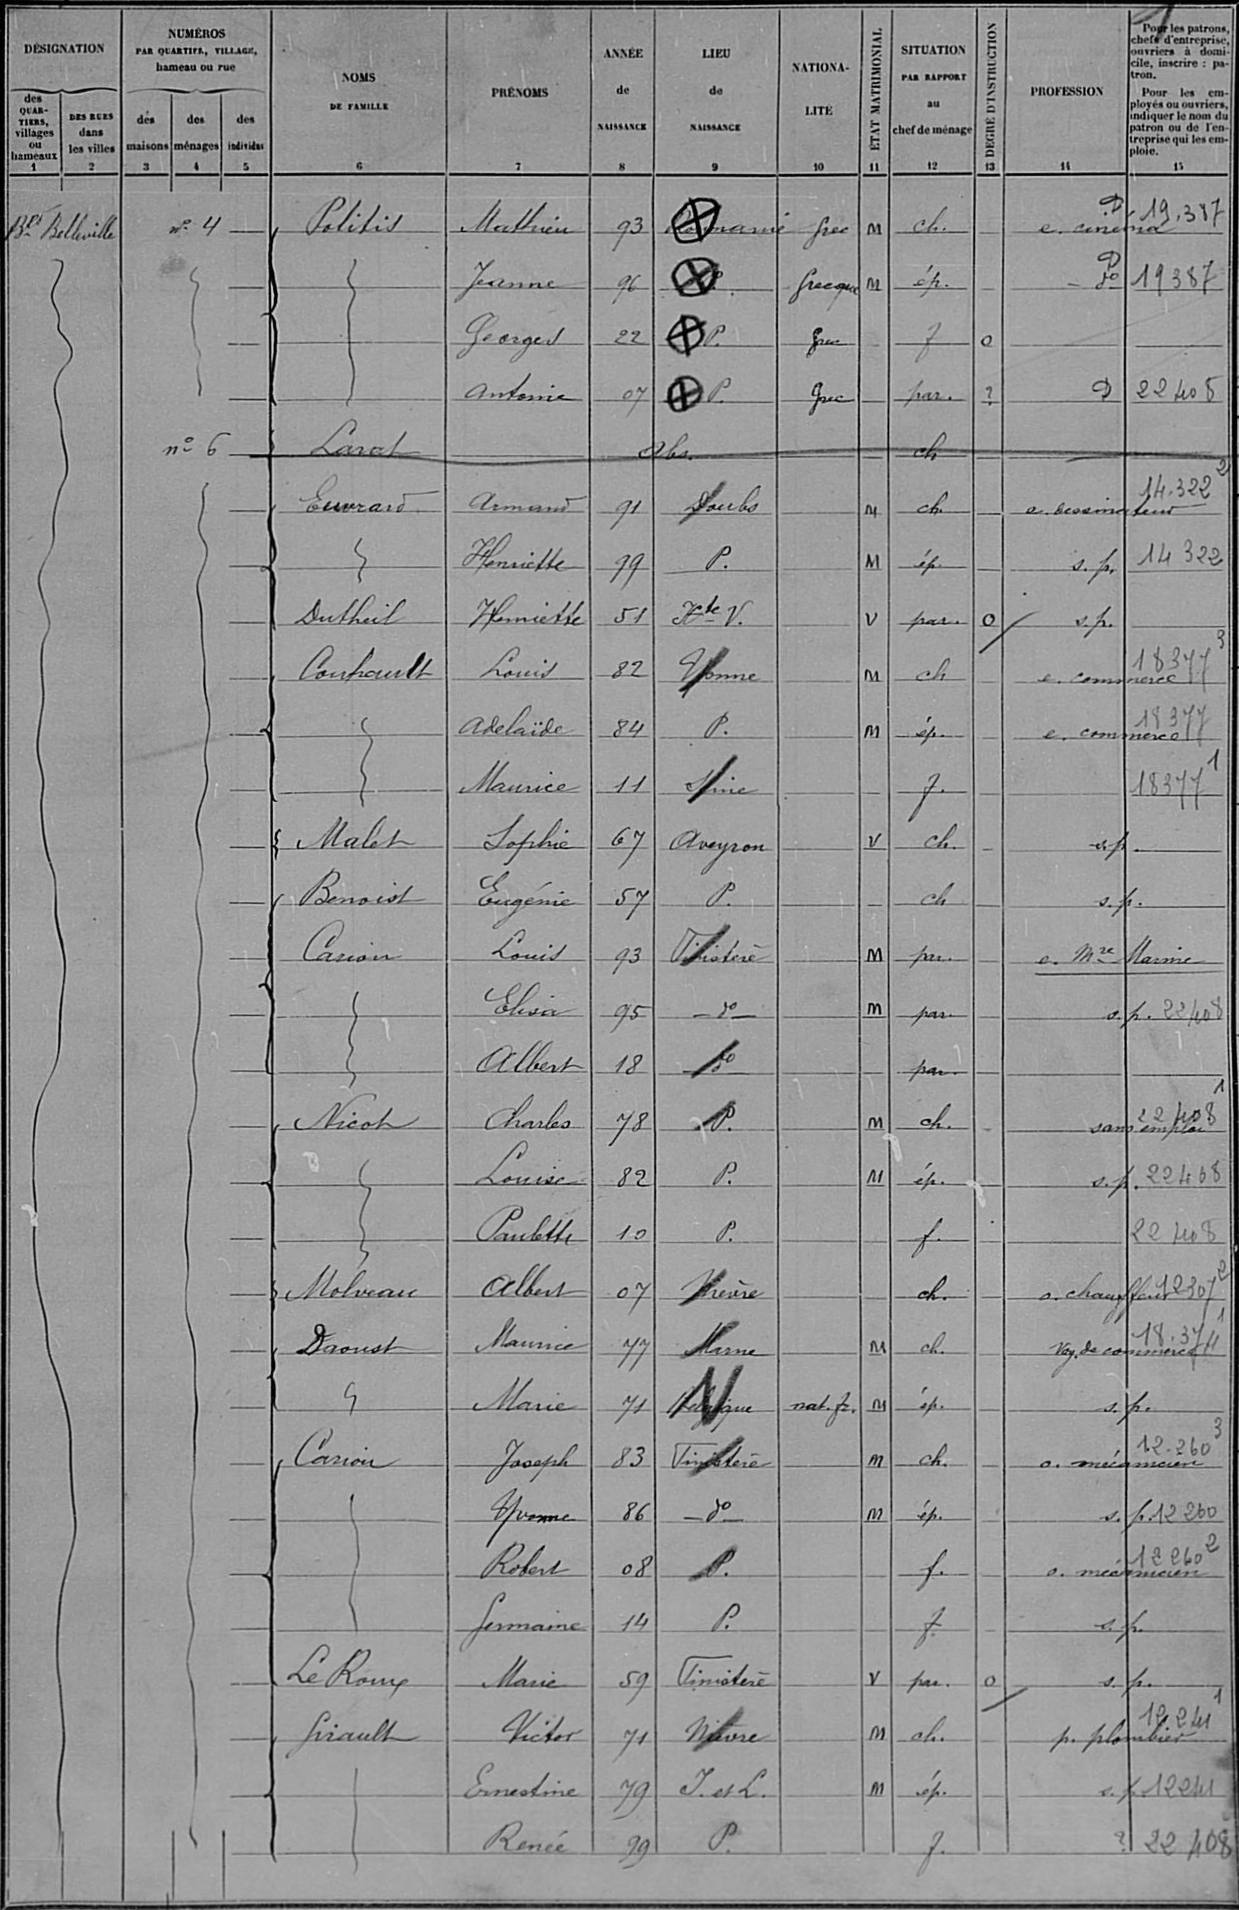Politis/Mathieu/93/Roumanie/grec/M/ch /¤/e cinéma/¤
¤/Jeanne/96/P /grecque/M/ép /¤/d°/19387
¤/Georges/22/P /grec/¤/f/o/¤/¤
¤/Antoine/07/P /grec/¤/par /¤/D/22408
Larat/¤/abs /¤/¤/¤/ch/¤/¤/¤
Euvrard/Armand/91/Doubs/¤/M/ch/¤/a dessinateur/¤
¤/Henriette/99/P /¤/M/ép /¤/s p/14322
Dutheil/Henriette/51/Hte V /¤/V/par /O/s p /¤
Couhault/Louis/82/Yonne/¤/M/ch/¤/e commerce/¤
¤/Adelaïde/84/P /¤/M/ép /¤/e commerce/¤
¤/Maurice/11/Seine/¤/¤/f /¤/¤/18377
Malet/Sophie/67/Aveyron/¤/V/ch/¤/s p /¤
Benoist/Eugénie/57/P /¤/¤/ch/¤/s p /¤
Carion/Louis/93/Finistère/¤/M/par /¤/e Mre Marine/¤
¤/Elisa/95/d°/¤/M/par /¤/s p /22408
¤/Albert/18/d°/¤/¤/par /¤/¤/¤
Nicoh/Charles/78/P /¤/M/ch /¤/sans emploi/¤
¤/Louise/82/P /¤/M/ép /¤/s p /22408
¤/Paulette/10/P /¤/¤/f /¤/¤/22408
Molvearc/Albert/07/Nièvre/¤/¤/ch /¤/o chauffeur/12307
Daoust/Maurice/77/Marne/¤/M/ch /¤/voy de commerce/¤
¤/Marie/71/Belgique/nat fr /M/ép/¤/s p /¤
Cariou/Joseph/83/Finistère/¤/M/ch /¤/o mécanicien/¤
¤/Yvonne/86/d°/¤/m/ép /¤/s p /12260
¤/Robert/08/P /¤/¤/f /¤/o mécanicien/¤
¤/Germaine/14/P /¤/¤/f/¤/s p /¤
Le Roux/Marie/59/Finistère/¤/V/par /o/s p /¤
Girault/Victor/71/Nièvre/¤/M/ch /¤/p plombier/¤
¤/Ernestine/79/I et L /¤/M/ép /¤/s p /12241
¤/Renée/99/P /¤/¤/f /¤/¤/22408
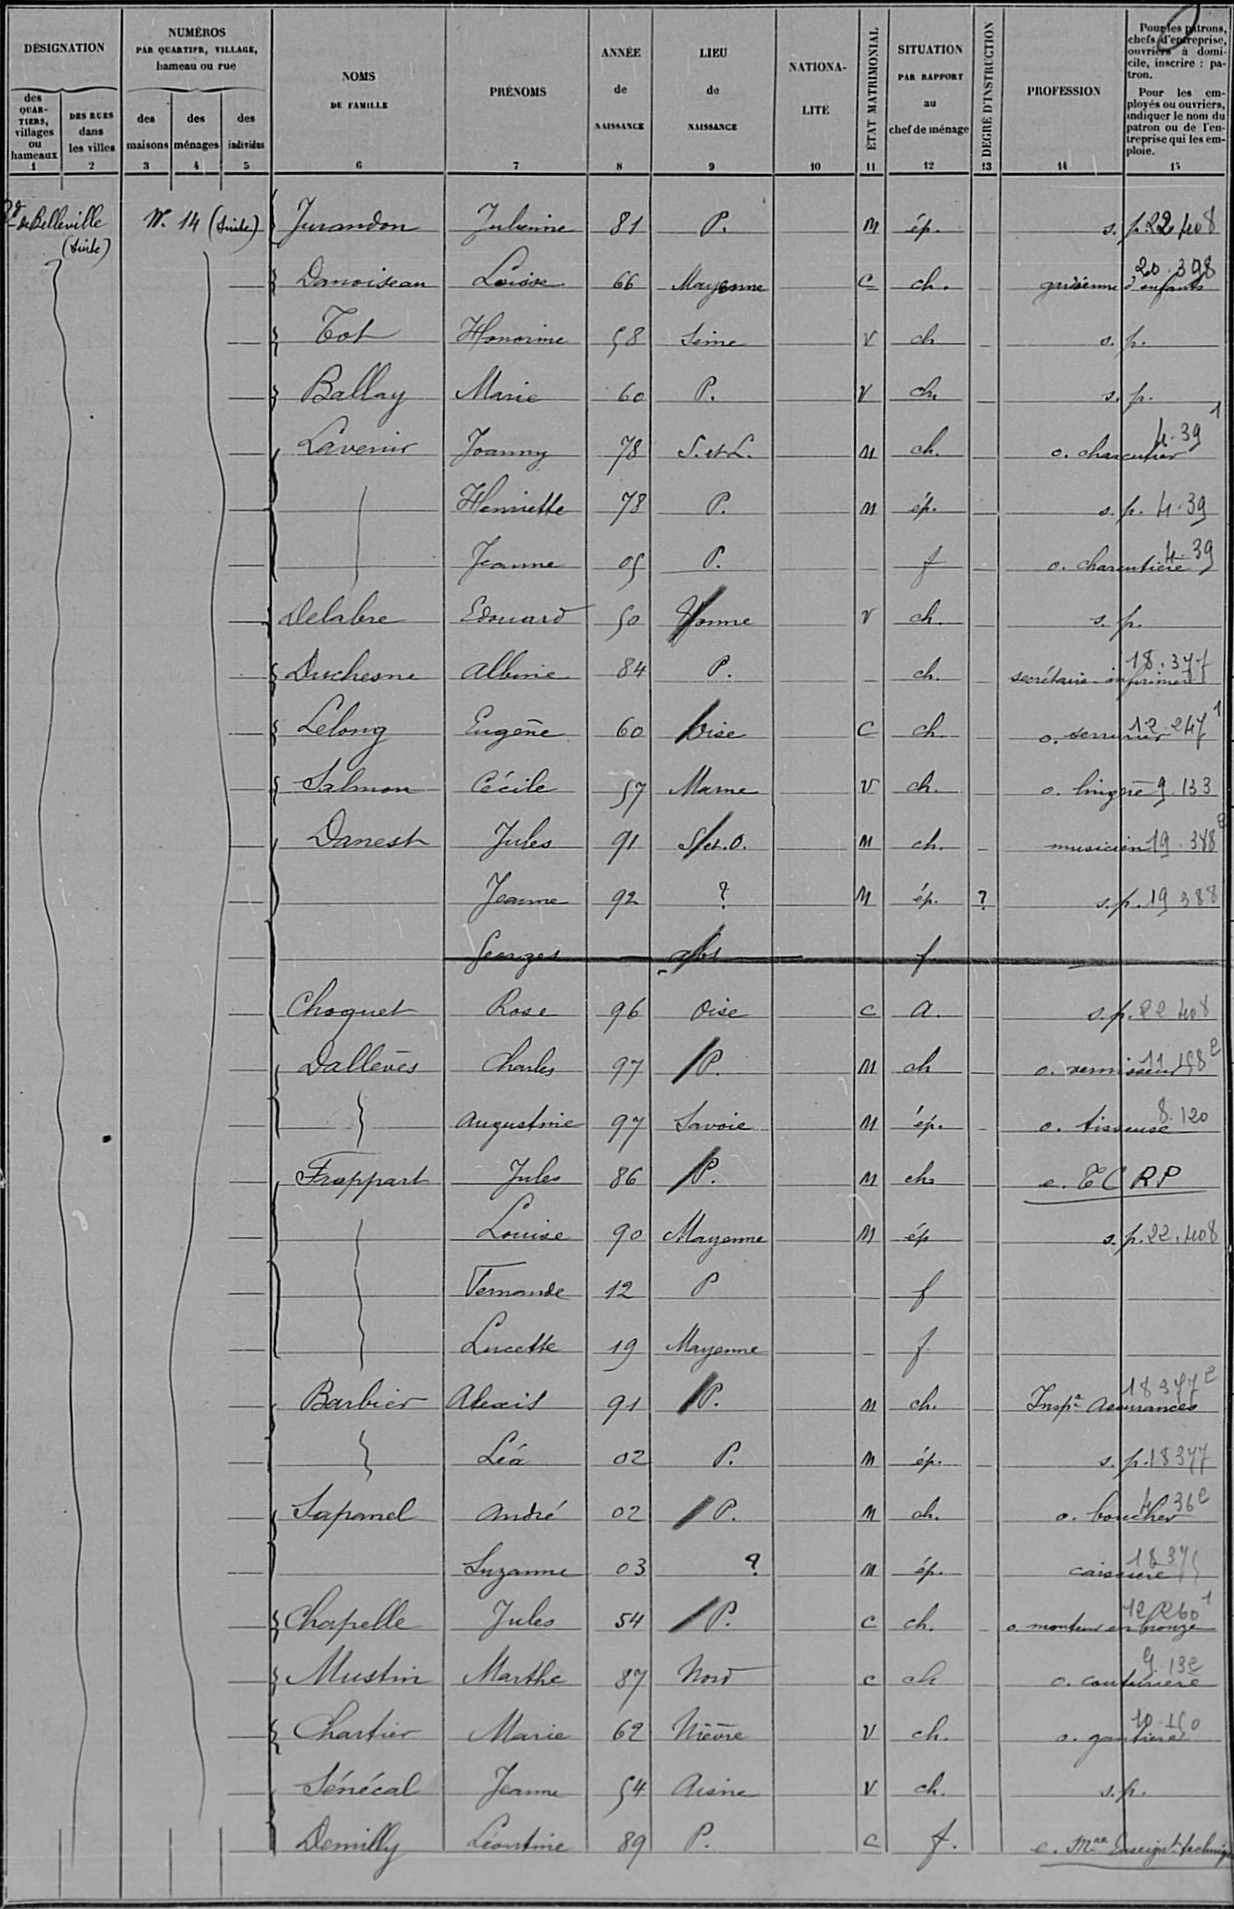Jurandon/Julienne/81/P /¤/M/ép /¤/s p /22408
Damoiseau/Louise/66/Mayenne/¤/C/ch /¤/gardienne d'enfants/¤
Tot/Honorine/58/Seine/¤/V/ch/¤/s p /¤
Ballay/Marie/60/P /¤/V/ch /¤/s p /¤
Lavenir/Joanny/78/S et L /¤/M/ch /¤/o charcutier/¤
¤/Henriette/78/P /¤/M/ép /¤/s p /4 39
¤/Jeanne/05/P /¤/¤/f/¤/o charcutiere/39
Delabre/Edouard/50/Somme/¤/V/ch/¤/s p /¤
Duchesne/Albine/84/P /¤/¤/ch /¤/secrétaire infirmière/¤
Lelong/Eugène/60/Oise/¤/C/ch/¤/o serrurier/12 247
Salmon/Cécile/57/Marne/¤/V/ch /¤/o lingère/9 133
Danest/Jules/91/S et O/¤/M/ch /¤/musicien/19 388
¤/Jeanne/92/¤/¤/M/ép /¤/s p /19388
¤/Georges/¤/abs/¤/¤/f/¤/¤/¤
Choquet/Rose/96/Oise/¤/c/a /¤/s p /22408
Dallènes/Charles/97/P /¤/M/ch/¤/o vernisseur/¤
¤/Augustine/97/Savoie/¤/M/ép /¤/o tisseuse/¤
Frappart/Jules/86/P /¤/M/ch /¤/e TCRP/¤
¤/Louise/90/Mayenne/¤/M/ép/¤/s p /22 408
¤/Fernande/12/P/¤/¤/f/¤/¤/¤
¤/Lucette/19/Mayenne/¤/¤/f/¤/¤/¤
Barbier/Alexis/91/P /¤/M/ch /¤/Insp assurances/¤
¤/Léa/02/P /¤/M/ép /¤/s p /18377
Sapanel/André/02/P /¤/M/ch /¤/o boucher/¤
¤/Suzanne/03/¤/¤/m/ép /¤/caissière/¤
Chapelle/Jules/54/P /¤/c/ch /¤/o mouleur en bronze/¤
Mustin/Marthe/87/Nord/¤/c/ch/¤/o couturière/¤
Chartier/Marie/62/Nièvre/¤/V/ch /¤/o gantiere/¤
Sénécal/Jeanne/54/Aisne/¤/V/ch /¤/s p /¤
Demilly/Léontine/89/P /¤/c/f /¤/e M enseignt techniqu/¤
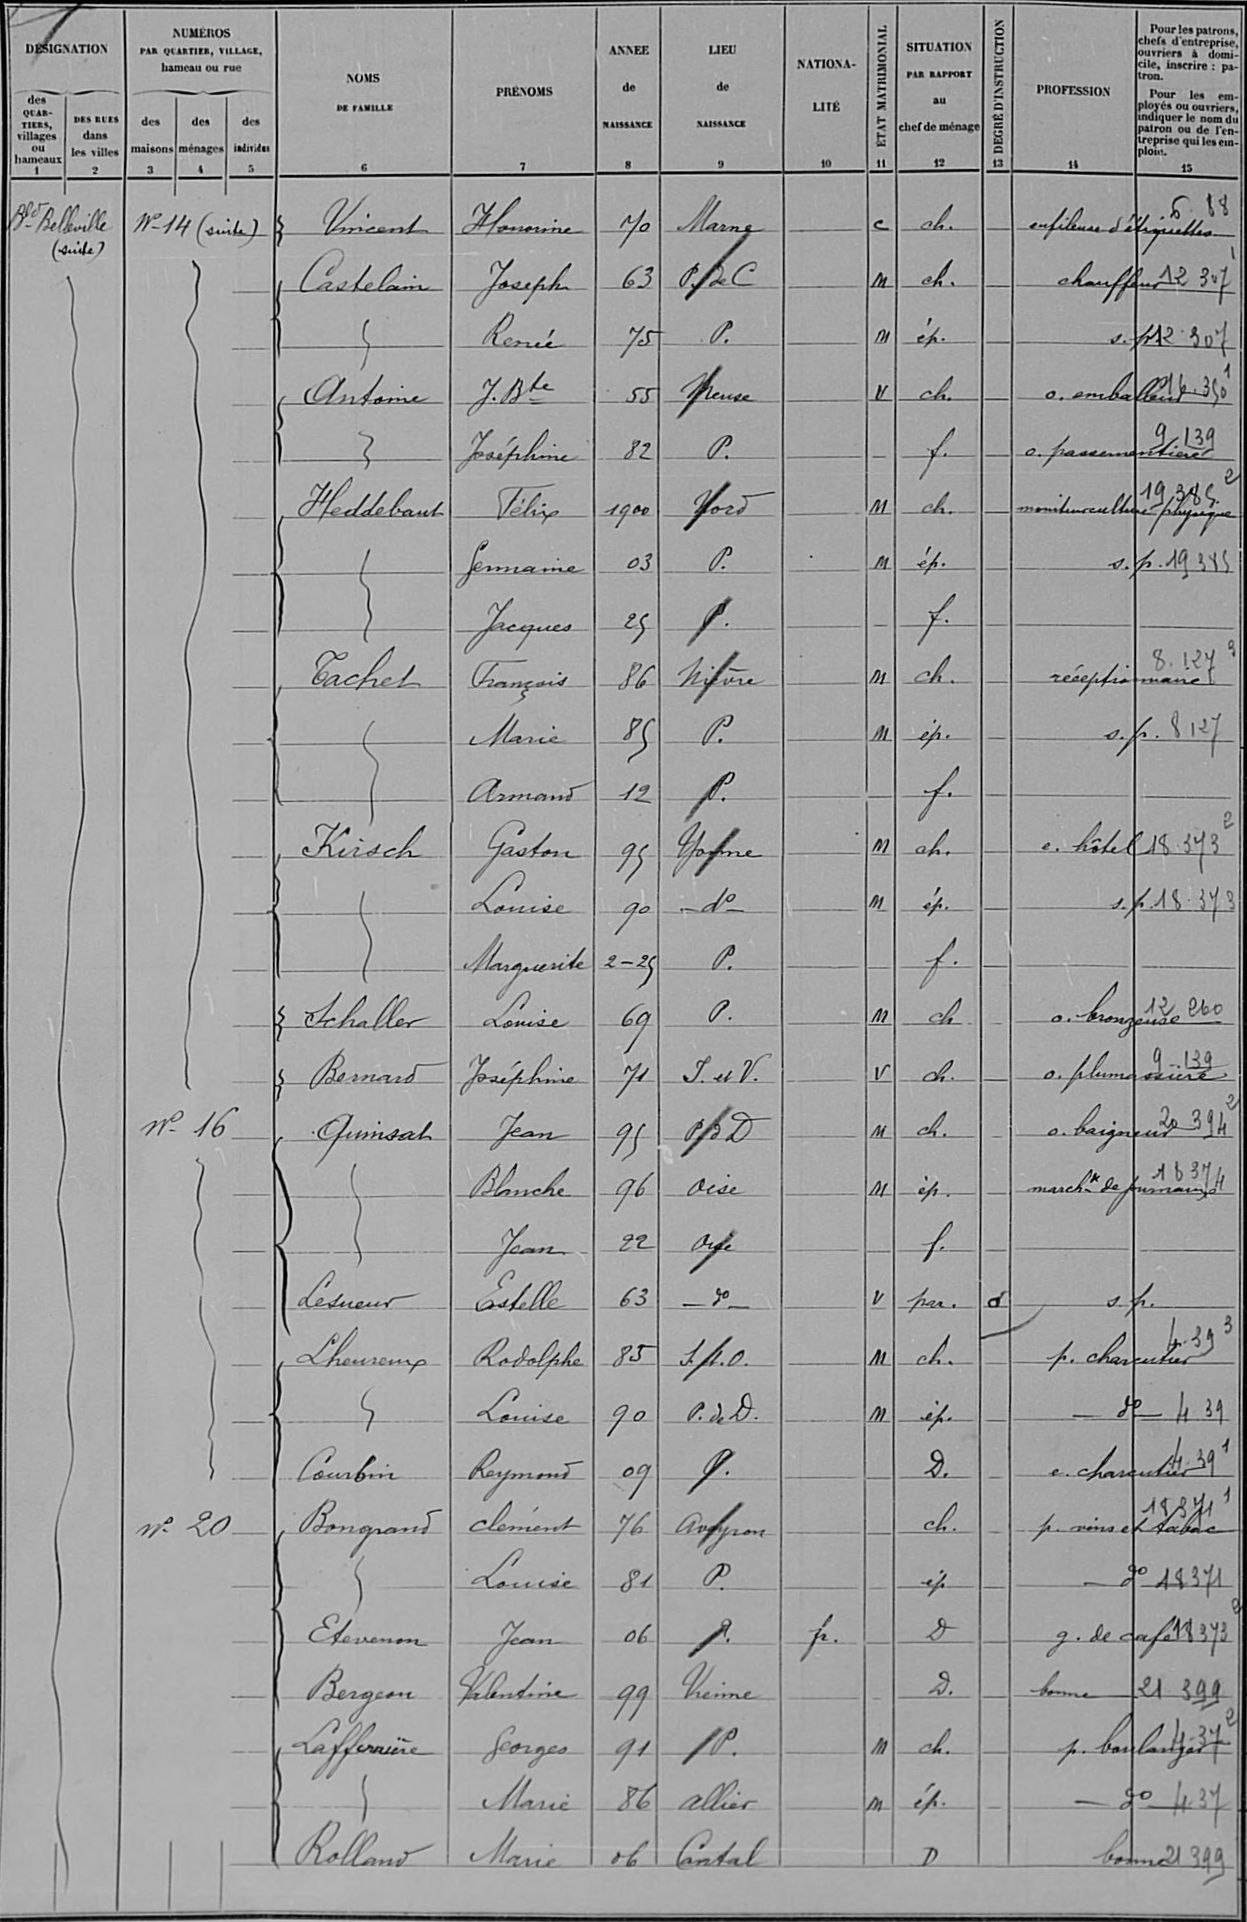Vincent/Honorine/Mo/Marne/¤/c/ch /¤/enfileuse d'étiquettes/¤
Castelain/Joseph/63/P de C/¤/M/ch /¤/chauffeur/12307
¤/Renée/75/P /¤/m/ép /¤/s pr /12307
Antoine/J Bte/55/Meuse/¤/V/ch /¤/o emballeur/16 3501
¤/Joséphine/82/P /¤/¤/f /¤/o passementiere/¤
Heddebaut/Félix/1900/Nord/¤/M/ch /¤/moniteur culture/physique
¤/Germaine/03/P /¤/M/ép /¤/s /pr 19385
¤/Jacques/25/P /¤/¤/f /¤/¤/¤/¤
Cachet/François/86/Nièvre/¤/M/ch /¤/réceptionnaire/¤
¤/Marie/85/P /¤/M/ép /¤/s /pr 8127
¤/Armand/12/P /¤/¤/f /¤/¤/¤/¤
Kirsch/Gaston/95/Yonne/¤/M/ch /¤/e hôtel/183732
¤/Louise/90/d°/¤/M/ép /¤/s /pr 18373
¤/marguerite/2 25/P /¤/¤/f /¤/¤/¤/¤
Schaller/Louise/69/P /¤/M/ch/¤/o bronzeuse/12260
Bernard/Joséphine/71/I et V /¤/V/ch /¤/o plumassière/¤
Quinsat/Jean/95/P d D/¤/M/ch /¤/o baigneur/203942
¤/Blanche/96/Oise/¤/M/ép /¤/marchd de journaux/¤
¤/Jean/22/Oise/¤/¤/f /¤/¤/¤/¤
Lesueur/Estelle/63/d°/¤/V/par /o/s/pr
Lheureux/Rodolphe/85/S et O /¤/M/ch /¤/p charcutier/¤
¤/Louise/90/P de D /¤/M/ép /¤/d°/439
Courbin/Raymond/09/P /¤/¤/D /¤/e charcutier/4391
Bongrand/Clément/76/Aveyron/¤/¤/ch /¤/p vins et tabac/¤
¤/Louise/81/P /¤/¤/ép/¤/d°/18371
Etevenon/Jean/06/¤/fr /¤/D/¤/g de café/18373
Bergeon/Valentine/99/Vienne/¤/¤/D /¤/bonne/21399
Lefferrière/Georges/91/P /¤/M/ch /¤/p boulanger/4372
¤/Marie/86/allier/¤/m/ép /¤/d°/437
Rolland/Marie/06/Cantal/¤/¤/D/¤/bonne/21399